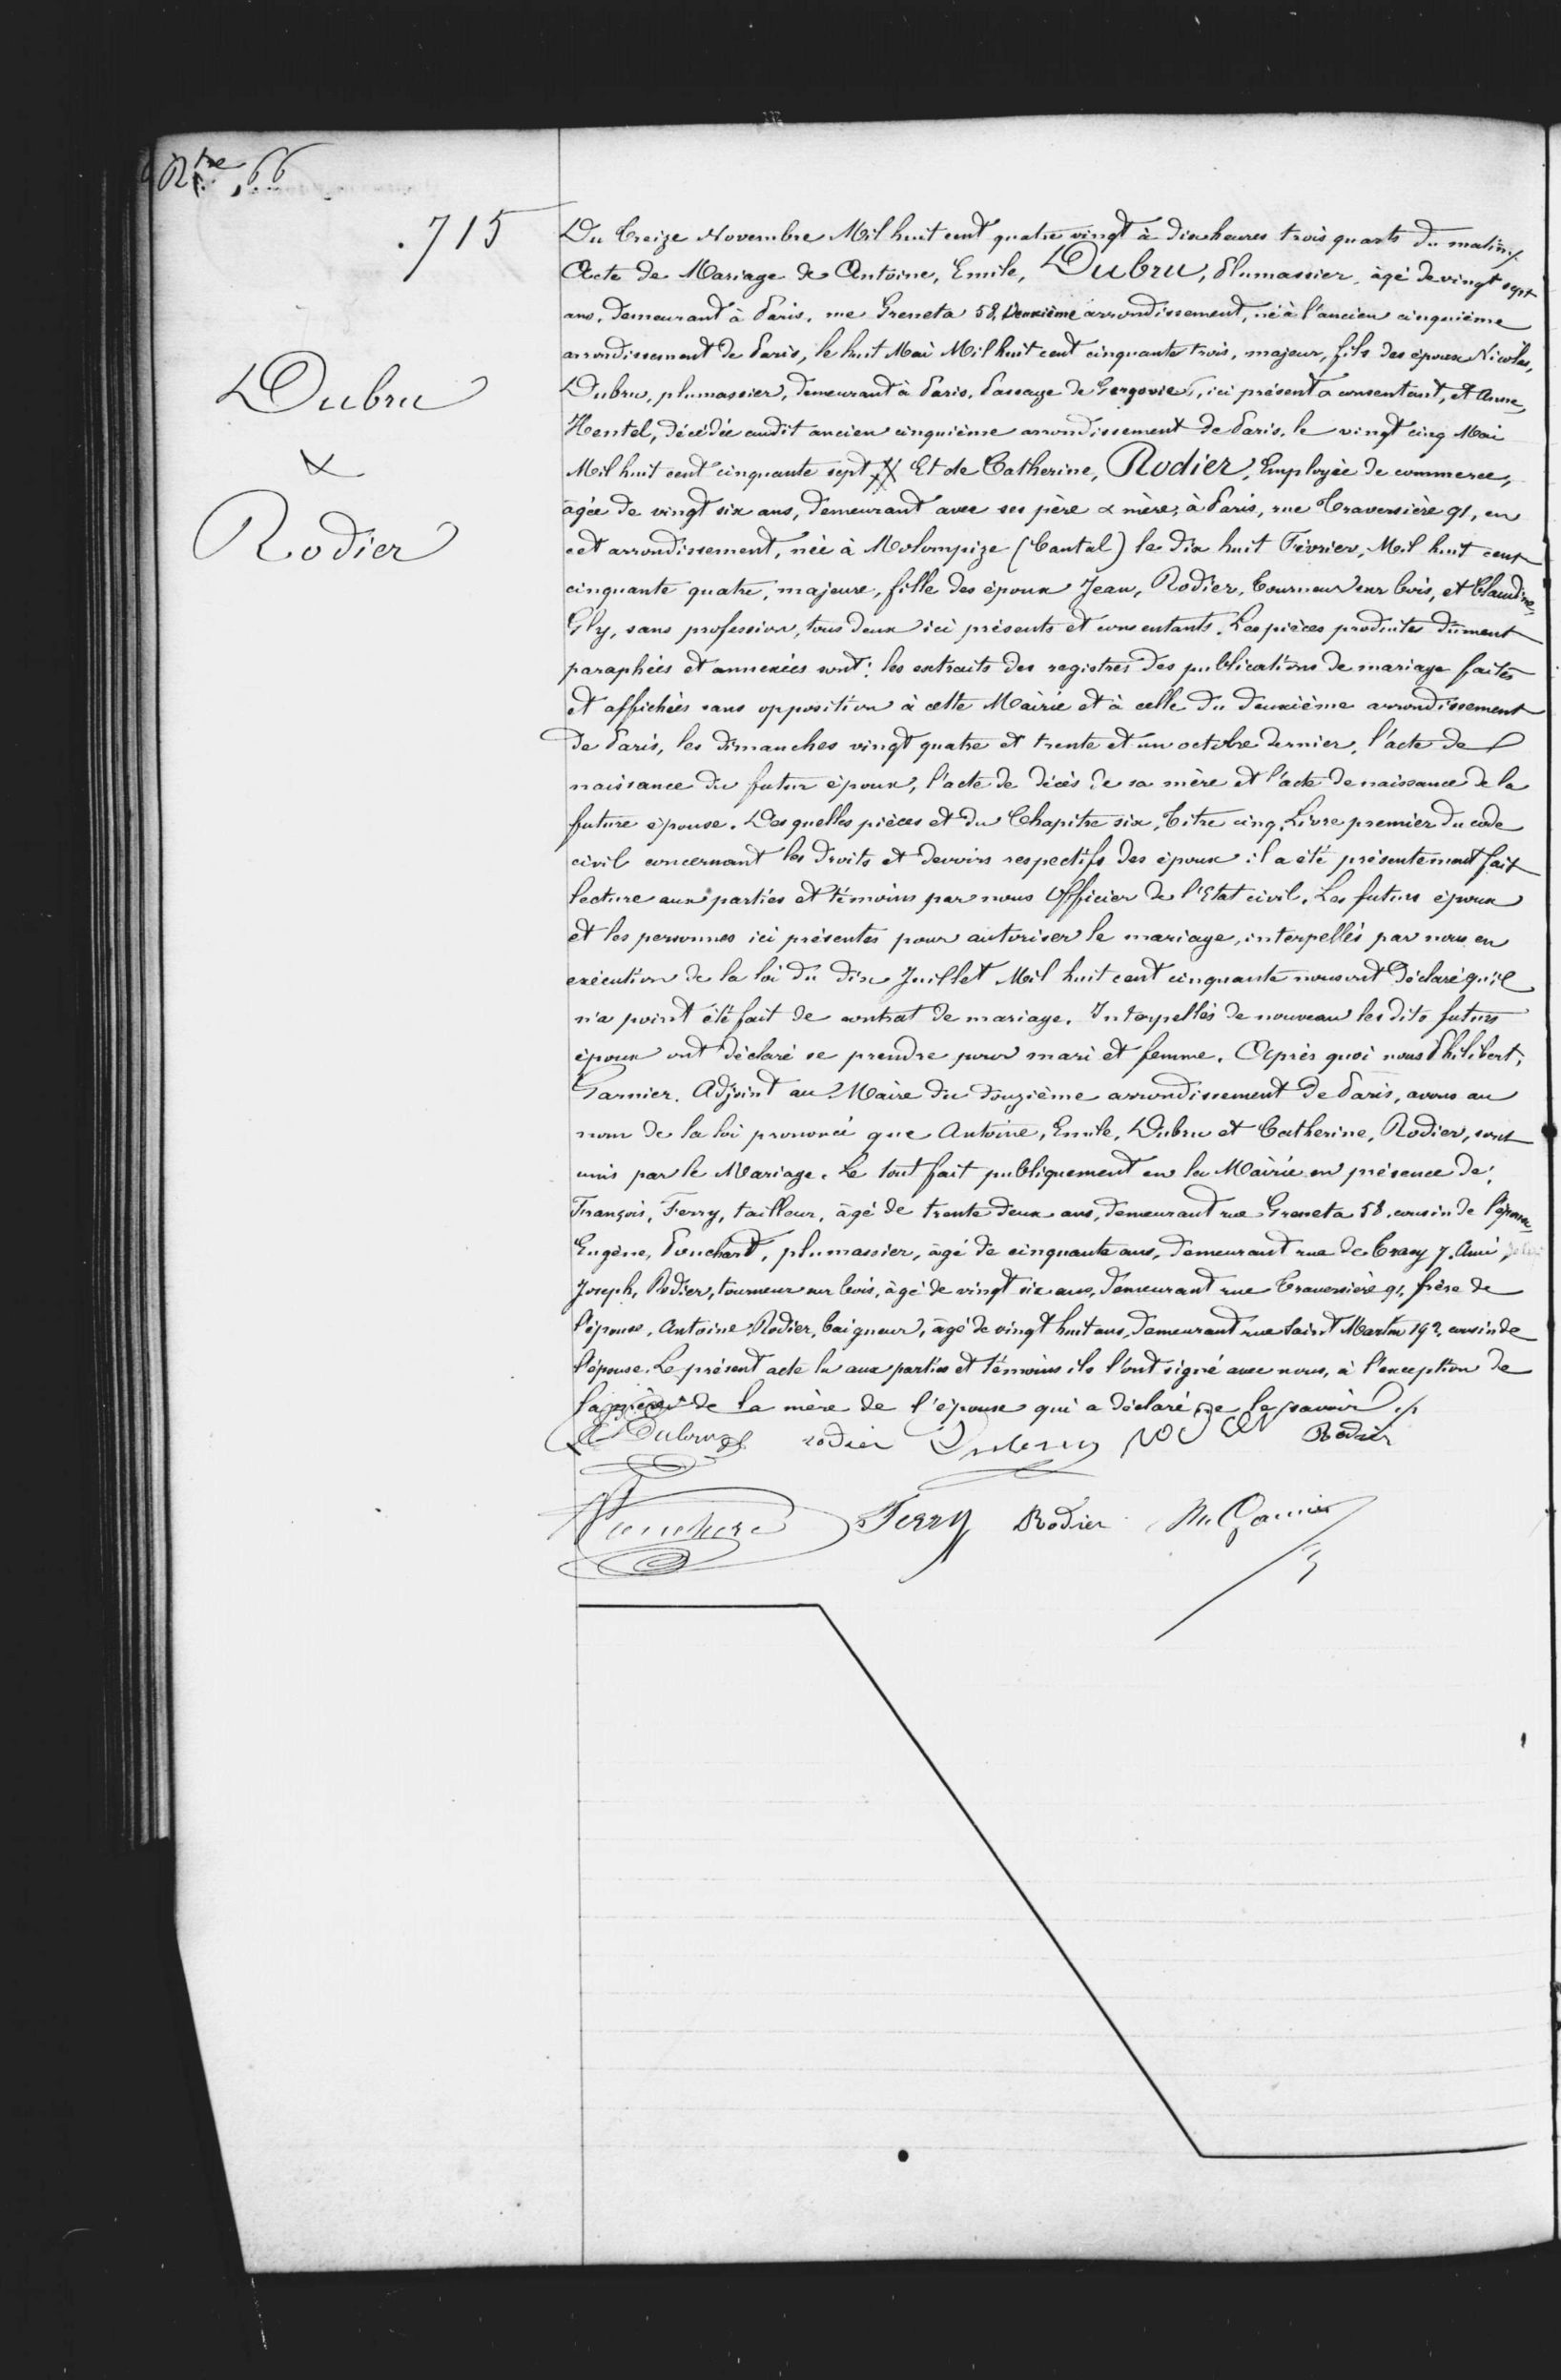715
Dubru
&
Rodier
Du Treize Novembre Mil huit cent, quatre vingt à dix heures trois quarts du matin ./.
Acte de Mariage de Antoine, Emile, Dubru, plumassier, âgé de vingt sept
ans, demeurant à Paris, rue Greneta 58, Deuxième arrondissement, né à l'ancien cinquième
arrondissement de Paris, le huit Mai Mil huit cent cinquante trois, majeur, fils des époux Nicolas,
Dubru, plumassier, demeurant à Paris, Passage de Gergovie, ici présent et consentant, et Anne
Hentel, décédée audit ancien cinquième arrondissement de Paris, le vingt cinq Mai
Mil huit cent cinquante sept # Et de Catherine Rudier, Employée de commerce,
âgée de vingt six ans, demeurant avec ses père et mère, à Paris, rue Traversière 98, en
cet arrondissement, née à Molompeize (Cantal) le dix huit Février, Mil huit cent
cinquante quatre, majeure, fille des époux Jean, Rodier, Tourneur sur bois, et Claudine
Gly, sans profession, tous deux ici présents et consentants. Les pièces produites dûment
paraphées et annexées sont: les extraits des registres des publications de mariage faites
et affichées sans opposition à cette Mairie et à celle du deuxième arrondissement
de Paris, les dimanches vingt quatre et trente et un octobre dernier, l'acte de
naissance du futur époux, l'acte de décès de sa mère et l'acte de naissance de la
future épouse. Desquelles pièces et du Chapitre six, Titre cinq, Livre premier du code
civil concernant les droits et devoirs respectifs des époux il a été présentement fait
lecture aux parties et témoins par nous Officier de l'Etat civil, Les futurs époux
et les personnes ici présentes pour autoriser le mariage, interpellés par nous en
exécution de la loi du dix Juillet Mil huit cent cinquante nous ont déclaré qu'il
n'a point été fait de contrat de mariage. Interpellés de nouveau les dits futurs
époux ont déclaré se prendre pour mari et femme. Après quoi nous Philibert,
Garnier, Adjoint au Maire du douzième arrondissement de Paris, avons au
nom de la loi prononcé que Antoine, Emile, Dubru et Catherine, Rodier, sont
unis par le Mariage. Le tout fait publiquement en la Mairie en présence de
François, Ferry, tailleur, âgé de trente deux ans, demeurant rue Greneta 58, cousin de l'épouse,
Eugène Fouchard, plumassier, âgé de cinquante ans, demeurant rue de Crasy 7, Ami,
Joseph, Rodier, tourneur sur bois, âgé de vingt six ans, demeurant rue Traverssière 91, frère de
l'épouse, Antoine Rodier,baigneur, âgé de vingt huit ans, demeurant rue Saint Martin 192, cousin de
l'épouse. Le présent acte lu aux parties et témoins ils l'ont signé avec nous, à l'exception de
la mère de la mère de l'épouse qui a déclaré ne le savoir ./.
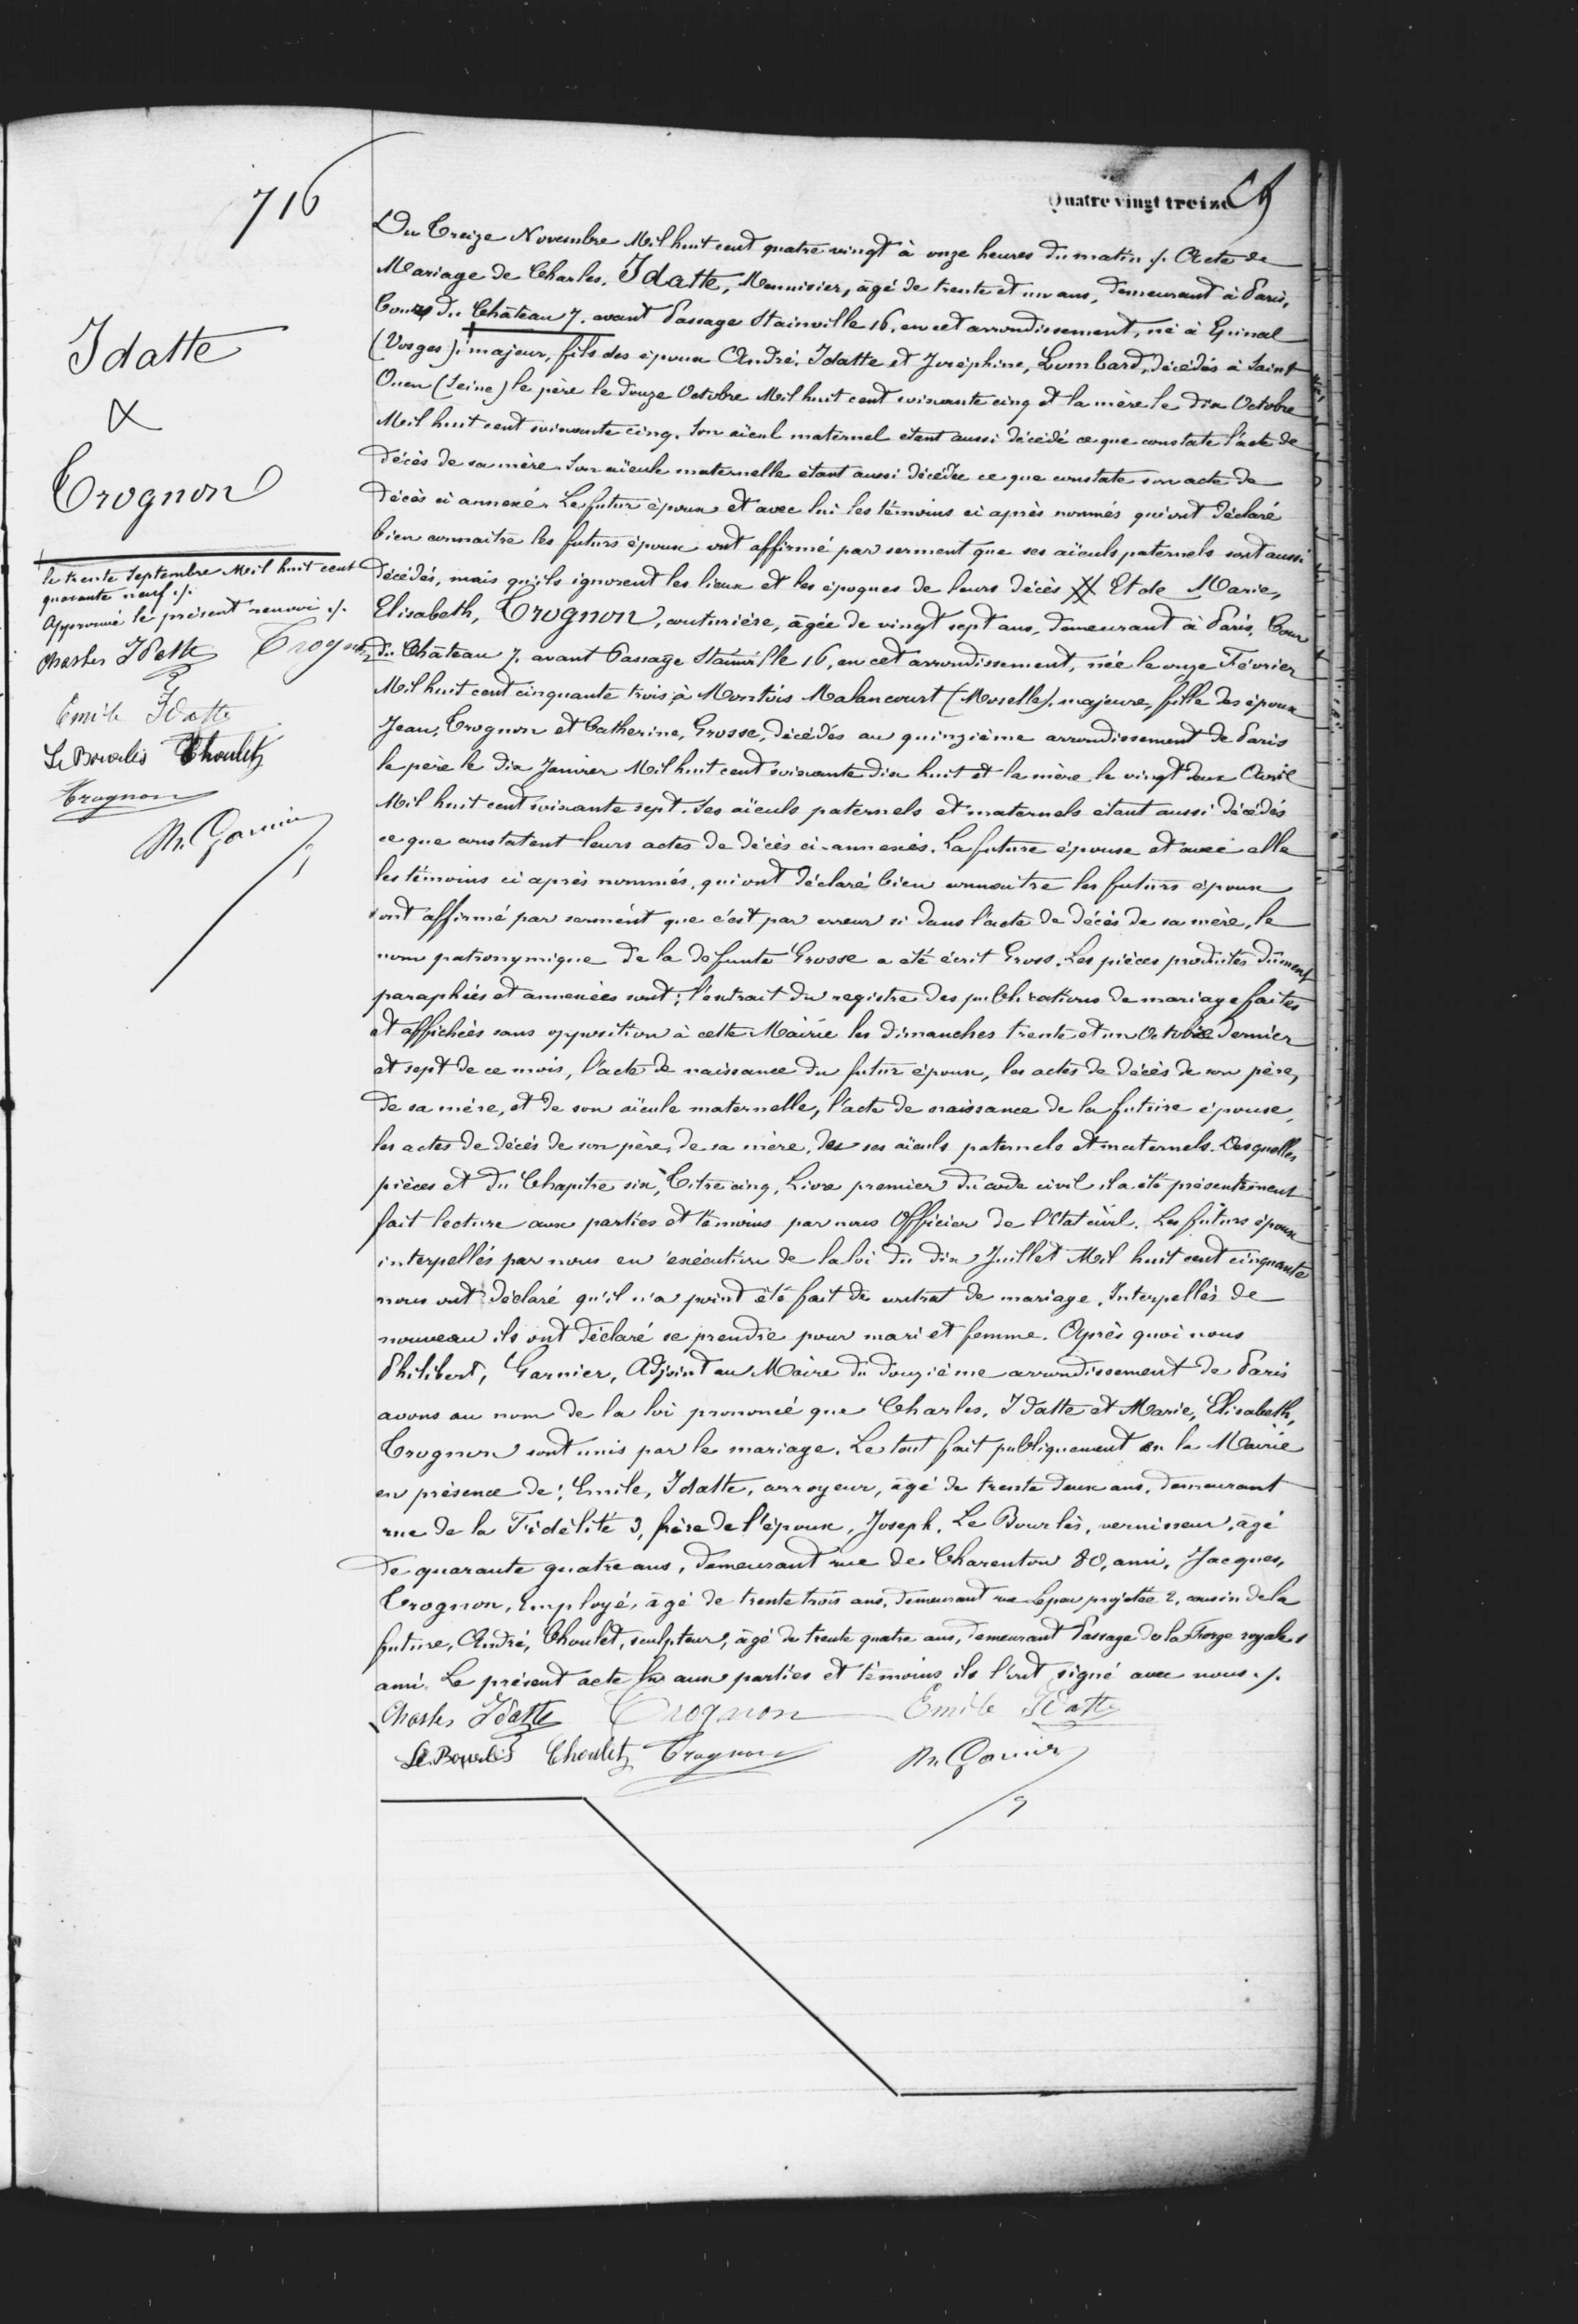716
Idatte
&
Trognon
Du Treize Novembre Mil huit cent quatre vingt à onze heures du matin ./. Acte de
Mariage de Charles, Idatte, Menuisier, âgé de trente et un ans, demeurant à Paris,
Cour du Château 7, avant Passage Atainville 16, en cet arrondissement né à Epinal
(Vosges) majeur, fils des époux André, Idatte et Joséphine, Lombard, décédés à Saint
Ouen (seine) le père le douze Octobre Mil huit cent soixante cinq et la mère le dix Octobre
Mil huit cent soixante cinq, son aïeul maternel étant aussi décédé ce que constate l'acte de
décès de sa mère, son aïeule maternelle étant aussi décédée ce que constate son acte de
décès ci annexé. Le futur époux et avec lui les témoins ci après nommés qui ont déclaré
bien connaître les futurs époux ont affirmé par serment que ses aïeuls paternels sont aussi
décédés, mais qu'ils ignorent les lieux et les époques de leur décès # Et de Marie,
Elisabeth, Trognon, couturière, âgée de vingt sept ans, demeurant à Paris, Cour
du Château ./. avant Passage Stainville 16, en cet arrondissement, née le onze Février
Mil huit cent cinquante trois à Montois Malancourt (Moselle), majeure, fille des époux
Jean, Trognon et Catherine, Grosse, décédés au quinzième arrondissement de Paris
le père le dix Janvier Mil huit cent soixante dix huit et la mère, le vingt deux Avril
Mil huit cent soixante sept. Ses aïeuls paternels et maternels étant aussi décédés
ce que constatent leurs actes de décès ci-annexés. La future épouse et avec elle
les témoins ci après nommés, qui ont déclaré bien connaître les futurs époux
ont affirmé par serment que c'est par erreur si dans l'acte de décès de sa mère, le
nom patronymique de la defunte Grosse a été écrit Gross. Les pièces produites dûment
paraphées et annexées sont: l'extrait du registre des publications de mariage faites
et affichées sans opposition à cette Mairie les dimanches trente et un Octobre dernier
et sept de ce mois, l'acte de naissance du futur époux, les actes de décès de son père,
de sa mère, et de son aïeule maternelle, l'acte de naissance de la future épouse
les actes de décès de son père, de sa mère, de ses aïeuls paternels et maternels. Desquelles
pièces et du Chapitre six, Titre cinq, Livre premier du code civil il a été présentement
fait lecture aux parties et témoins par nous Officier de l'Etat civil. Les futurs époux
interpellés par nous en éxécution de la loi du dix Juillet Mil huit cent cinquante
nous ont déclaré qu'il n'a point été fait de contrat de mariage. Interpellés de
nouveau ils ont déclaré se prendre pour mari et femme. Après quoi nous
Philibert, Garnier, Adjoint au Maire du douzième arrondissement de Paris
avons au nom de la loi prononcé que Charles, Idatte et Marie, Elisabeth,
Trognon sont unis par le mariage. Le tout fait publiquement en la Mairie
en présence de; Emile, Idatte, arroyeur, âgé de trente deux ans, demeurant
rue de la Fidèlité 9, frère de l'époux, Joseph, Le Bourlès, vernisseur, âgé
de quarante quatre ans, demeurant rue de Charenton 80, ami, Jacques,
Trognon, Employé, âgé de trente trois ans, demeurant rue Lepeu projetée 2, cousin de la
future, André, Choulet, sculpteur, âgé de trente quatre ans, demeurant Passage de la Forge royale,
ami. Le présent acte lu aux parties et témoins ils l'ont signé avec nous ./.
Charles Idatte Trognon Emile Idatte
Le Bourlès Choulet Trognon Du Garnier